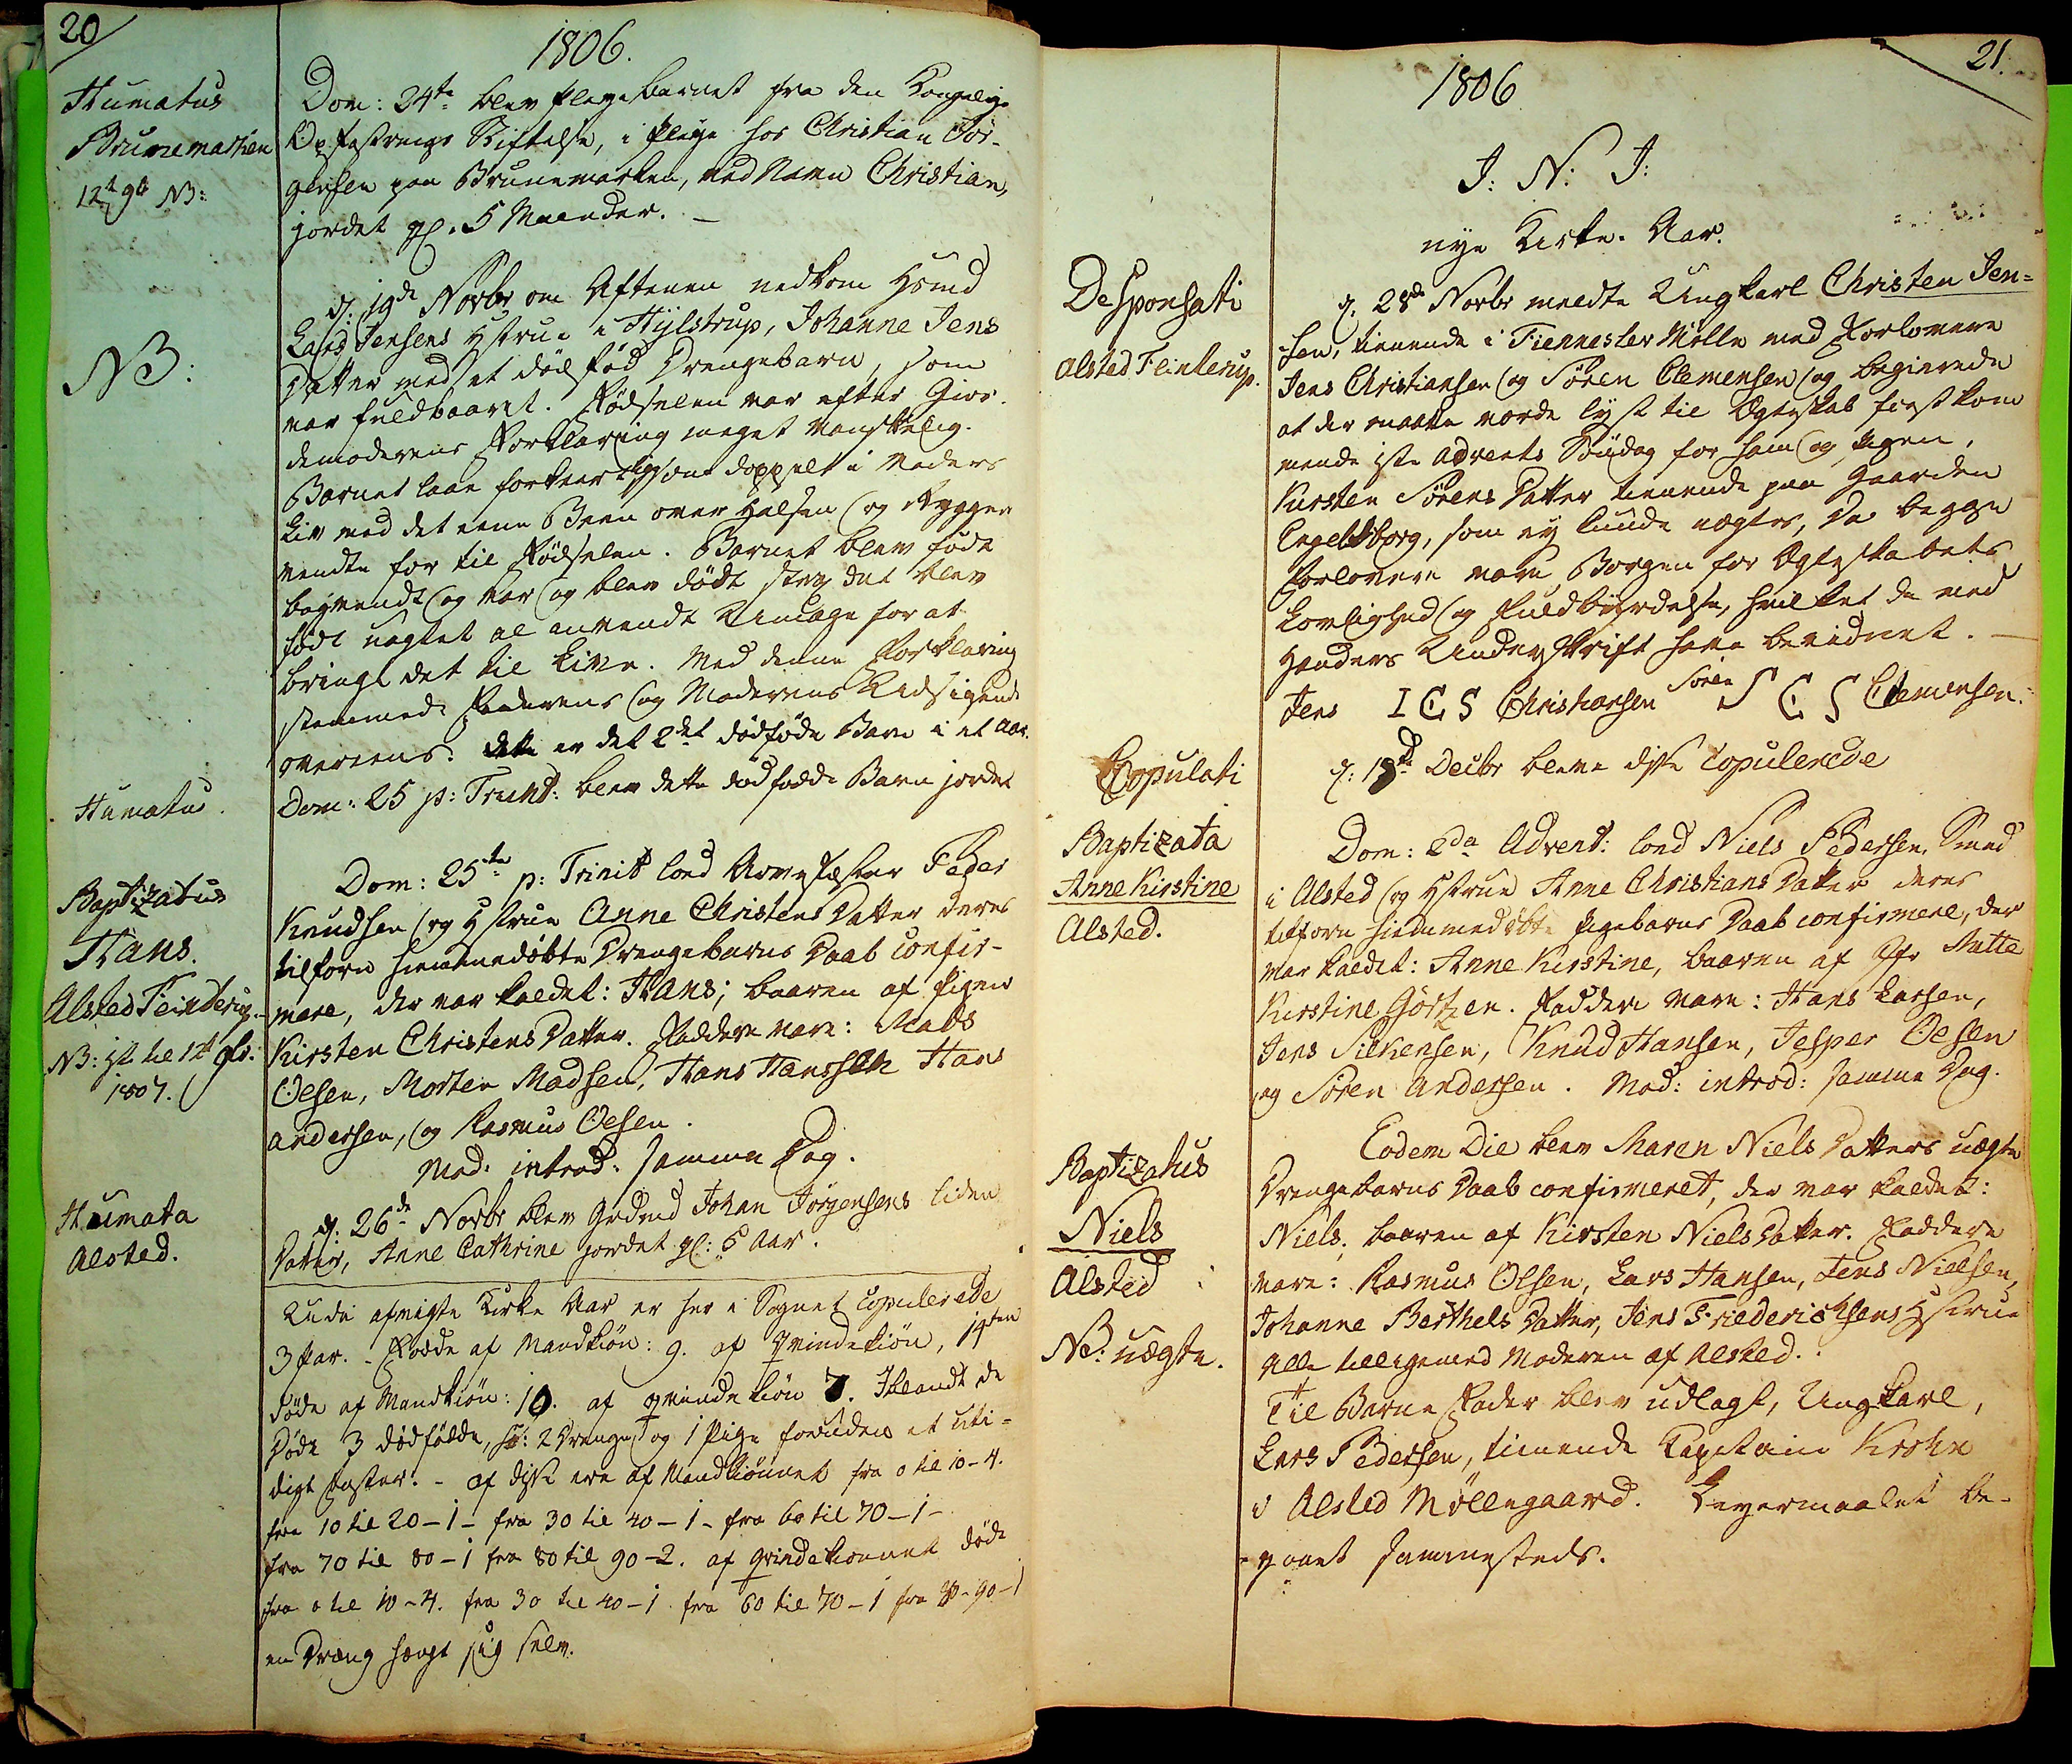20
Humatus
Brunemarken
12te  9## NB:
NB:
Humata.
Baptizatus
Hans
Alsted Flinterup.
NB: 1ste til 12t 9br.
1807.
Humata
Alsted
1806
Dom: 24ta blev Plegebarnet fra den Kongelige
Opfostrings Stiftelse, i Plege hos Christian Jør¬
gensen paa Brunemarken, ved Navn Christian
jordet gl. 5 Maaneder.
d: 19de Novbr: om Aftenen nedkom Hsmd
Lars Jensens Hstrue i Hylstrup, Johanne Jens
Datter med et dødfød Drengebarn, som
var fuldbaaret. Fødselen var efter Gior¬
demoderens Forklaring meget vanskelig.
Barnet laae forkert ## doppelt i Moders
Liv med det eene Been over Halsen og Ryggen
vendte for til Fødselen. Barnet blev født
bagvendt og var og blev død strax det blev
født uagtet at anvendt Umage for at
bringe det til Live. Med denne Forklaring
stemmede Faderens og Moderens Udsigende
overens. dette er det 2det dødføde Barn i et Aar.
Dom: 25 p: Trinit: blev dette dødfødde Barn jordet
Dom: 25ta p: Trinit loed Arvefæster Peder
Knudsen og Hstrue Anne Christens Datter deres
tilforn hiemmedøbte Drengebarns Daab confir¬
mere, der var kaldet: Hans; baaren af Pigen
Kirsten Christens Datter. Faddere vare: Mads
Olsen, Morten Madsen, Hans Hanssen Hans
Andersen, og Rasmus Olsen
Mod: introd: samme Dag
d: 26de Novbr blev Grdmd Johan Jørgensens liden
Datter, Anne Cathrine jordet gl: 6 Aar.
Udi afvigte Kirke Aar er her i Sogne copulerede
3 Par. Fødde af Mandkiøn: 9. af Quindekiøn 14ten
døde af Mandkiøn: 10 af Qvindekiøn 7. Iblandt de
Døde 3 dødfødde, sc: 2 Drenge og 1 Pige foruden et uti¬
digt Foster. -  Af disse ere af Mandkiønnet fra 0 til 10 - 4
fra 10 til 20 - 1 - fra 30 til 40 - 1 - fra 60 til 70 - 1 -
fra 70 til 80 - 1 fra 80 til 90 - 2. af Qvindekiønnet døde
fra 0 til 10 - 4. fra 30 til 40 - 1 fra 60 til 70 - 1 fra 80 - 90 -1
en Dreng hængt sig selv.
Desponsati
Alsted Flinterup.
Copulati
Baptizata
Anne Kirstine
Alsted.
Baptizatus
Niels
Alsted
NB: uægte.
21.
1806
J: N: J:
nye Kirke. Aar.
d: 28de Novbr modte Ungkarl Christen Jen¬
sen, tienende i Fienneslev Mølle med Forlovere
Jens Christiansen og Søren Clemensen og begierede
at der maatte vorde lyst til Ægteskab forstkom
mende 1ste Advents Søndag for ham og Pigen,
Kirsten Sørens Datter tienende paa Gaarden
Engelsborg, som ey kunde nægtes, Da begge
Forlovere vare Borgen for Ægteskabets
Lovlighed og Fuldbyrdelse, hvilket de med
Hænders Underskrift have bevidnet.
Søren
Jens I C S Christiansen S C S Clemensen
d: 18De Decbr bleve disse copulerede
Dom: 2da Advent: loed Niels Pedersen Smed
i Alsted og Hstrue Anne Christians Datter deres
tilforn hiemmedøbt Pigebarns Daab confirmere, der
var kaldet: Anne Kirstine, baaren af Jfr Mette
Kirstine Gørtzen. Faddere vare: Hans Larsen,
Jens Silkensen, Knud Hansen, Jesper Olsen
og Søren Andersen. Mod: introd: samme Dag.
Eodem Die blev Maren Niels Datters uægte
Drengebarns Daab confirmeret, der var kaldet:
Niels. baaren af Kirsten Niels Datter. Faddere
vare: Rasmus Olsen, Lars Hansen, Jens Nielsen
Johanne Berthels Datter, Jens Friderichsens Hstrue
Alle tilligemed Moderen af Alsted.
Til Barne Fader blev udlagt, Ungkarl,
Lars Pedersen, tienende Kapetain Krohn
i Alsled Møllegaard. Leyermaalet be¬
gaaet sammesteds.
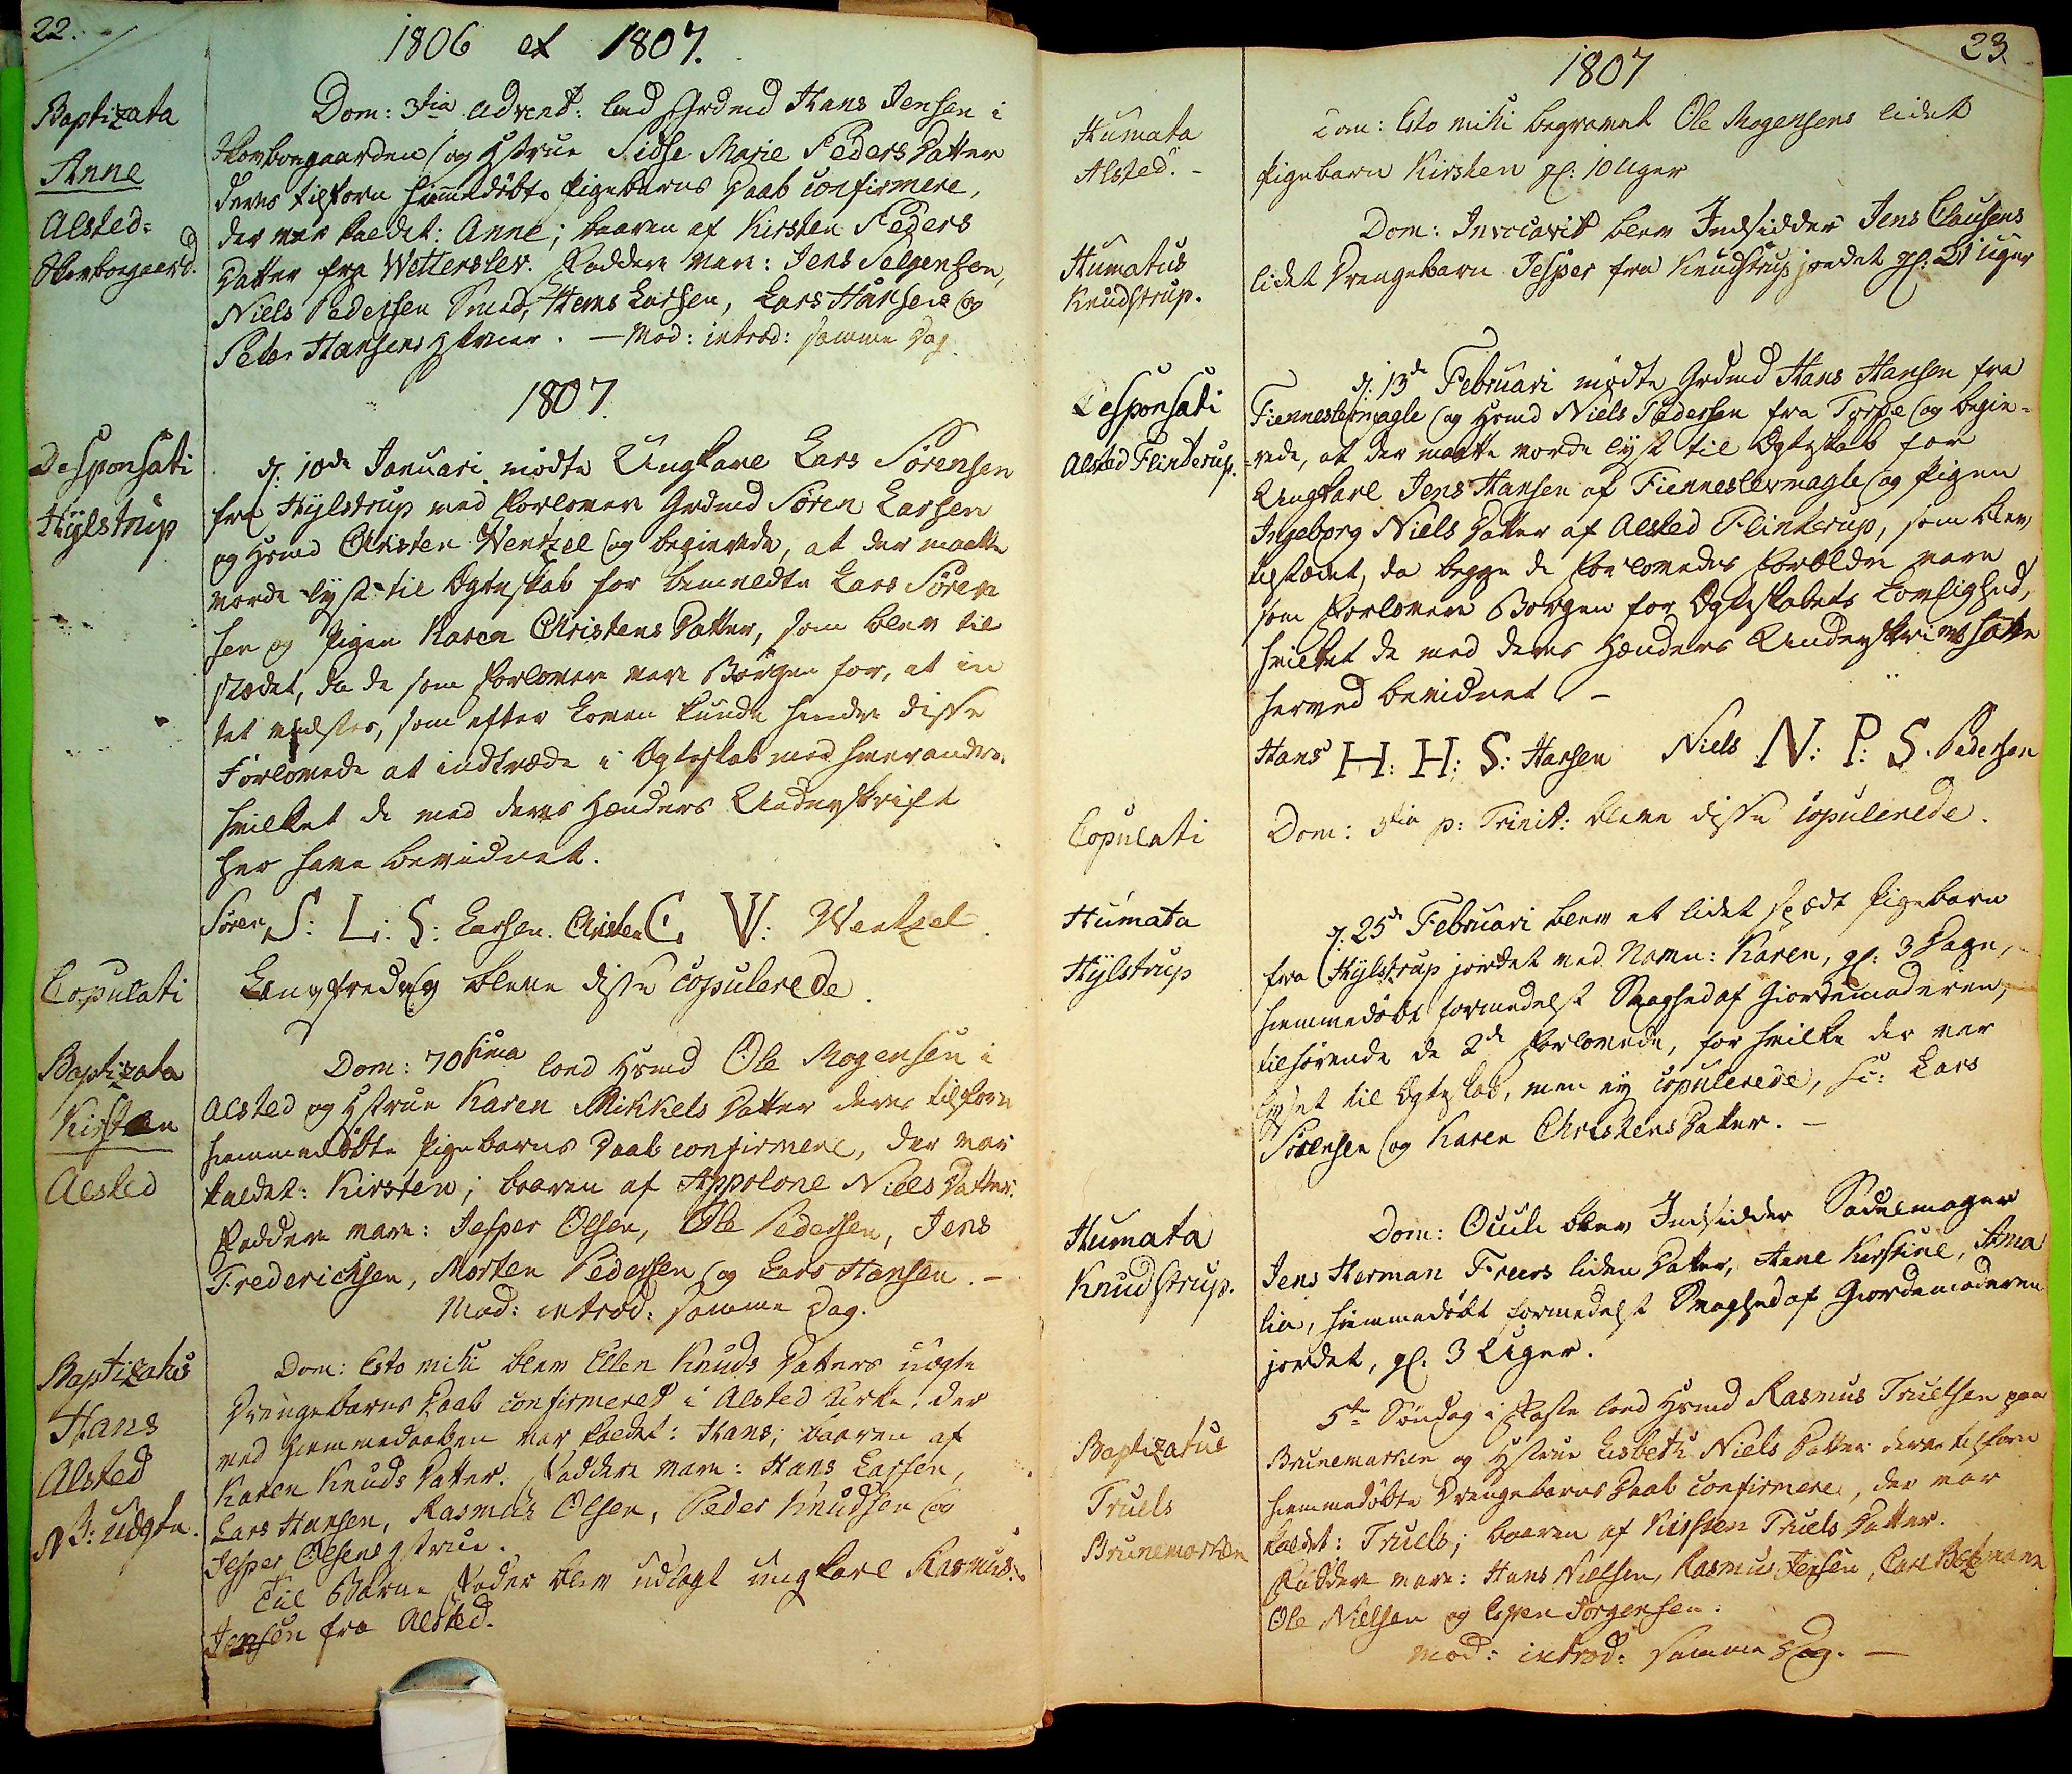22.
Baptizata
Anne
Alsted=
Skovboegaard.
Desponsati
Hylstrup
Copulati
Baptizata
Kristen
Alsted
Baptizatus
Hans
Alsted
NB: uægte.
1806 et 1807
Dom: 3tia Advent: loed Grdmd Hans Jensen i
Skovboegaarden og Hustrue Sidse Marie Peders Datter
deres tilforn hiemmedøbte Pigebarns Daab confirmere,
der var kaldet: Anne; baaren af Kirsten Peders
Datter fra Wetterslev. Faddere vare: Jens Selgensen
Niels Pedersen Smed, Hans Larsen, Lars Hansen og
Peter Hansens Hstrue. - Mod: introd: samme Dag
1807.
d: 10de Januari mødte Ungkarl Lars Sørensen
fra Hylstrup med Forlovere Grdmd Sørren Larsen
og Hsmd Christen Ventzel og begierede, at der maatte
vorde lyst til Ægteskab for bemeldte Lars Søren
sen og Pigen Karen Christens Datter, som blev til
stædet, da de som forlovere vare Borgen for at in
tet vidstes, som efter Loven kunde hindre disse
Forlovede at indræde i Ægteskab med hverandre.
hvilket de med deres Hænders Underskrift
her have bevidnet.
Søren S: L: S: Larsen Christen C W:  Wentzel
Langfredag bleve disse copulerede
Dom: 70sima loed Hsmd Ole Mogensen i
Alsted og Hstrue Karen Mikkels Datter deres tilforn
hiemmedøbte Pigebarns Daab confirmere, der var
kaldet: Kirsten; baaren af Appollone Niels Datter
Faddere vare: Jesper Olsen, Ole Pedersen, Jens
Frederichsen, Morten Pedersen og Lars Hansen
Mod: introd: samme Dag.
Dom: Esto mihi blev Ellen Knuds Datters uægte
Drengebarns Daab confirmeret i Alsted Kirke, der
ved Hiemmedaaben var kaldet: Hans; baaren af
Karen Knuds Datter. Faddere vare: Hans Larsen
Lars Hansen, Rasmus Olsen, Peder Knudsen og
Jesper Olsens Hstrue.
Til Barne Fader blev udlagt ungkarl Rasmus
Jensen fra Alsted.
Humata
Alsted.
Humatus
Knudstrup.
Desponsati
Alsted Flinterup.
Copulati
Humata
Hylstrup
Humata
Knudstrup.
Baptizatus
Truels
Brunemarken
23
1807
Dom: Esto mihi begravet Ole Mogensens lidet
Pigebarn Kirsten gl: 10 Uger
Dom: Invocavit blev Indsidder Jens Clausens
lidet Drengebarn Jesper fra Knudstrup jordet gl: 21 Uger
d: 13de Februari mødte Grdmd Hans Hansen fra
Finneslevmagle og Hsmd Niels Pedersen fra Torpe og begier¬
rede, at der maatte vorde lyst til Ægteskab for
Ungkarl Jens Hansen af Fienneslevmagle og Pigen
Ingeborg Niels Datter af Alsted Flinterup, som blev
tilstædet, da begge de forlovedes forældre vare
som Forlovere Borgen for Ægteskabets Lovlighed
hvilket de med deres Hænders Underskrivt have
herved bevidnet
Hans H: H. S: Hansen Niels N: P: S: Pedersen
Dom: 3tia p: Trinit: bleve disse copulerede.
d: 25de Februari blev et lidet spædt Pigebarn
fra Hylstrup jordet ved Navn: Karen, gl: 3 Dage,
hiemmedøbt formedelst Svaghed af Giordemoderen,
tilhørende de 2de Forlovede, for hvilke der var
Lyst til Ægteskab, men ey copulerede, sc: Lars
Sørensen og Karen Christens Datter.
Dom: Oculi blev Indsidder Sadelmager
Jens Herman Freers liden Datter, Anne Kirstine, Ama
lie, hiemmedøbt formedelst Svaghed af Giordemoderen
jordet gl: 3 Uger.
5te Søndag i Faste loed Hsmd Rasmus Truelsen paa
Brunemarken og Hustrue Lisbeth Niels Datter deres tilforn
hiemmedøbte Drengebarns Daab confirmeret, der var
kaldet: Truels; baaren af Kirsten Truels Datter
Faddere vare: Hans Nielsen, Rasmus Jensen, Carl Bætzmann
Ole Nielsen og Espen Jørgensen.
Mod: introd: samme Dag.